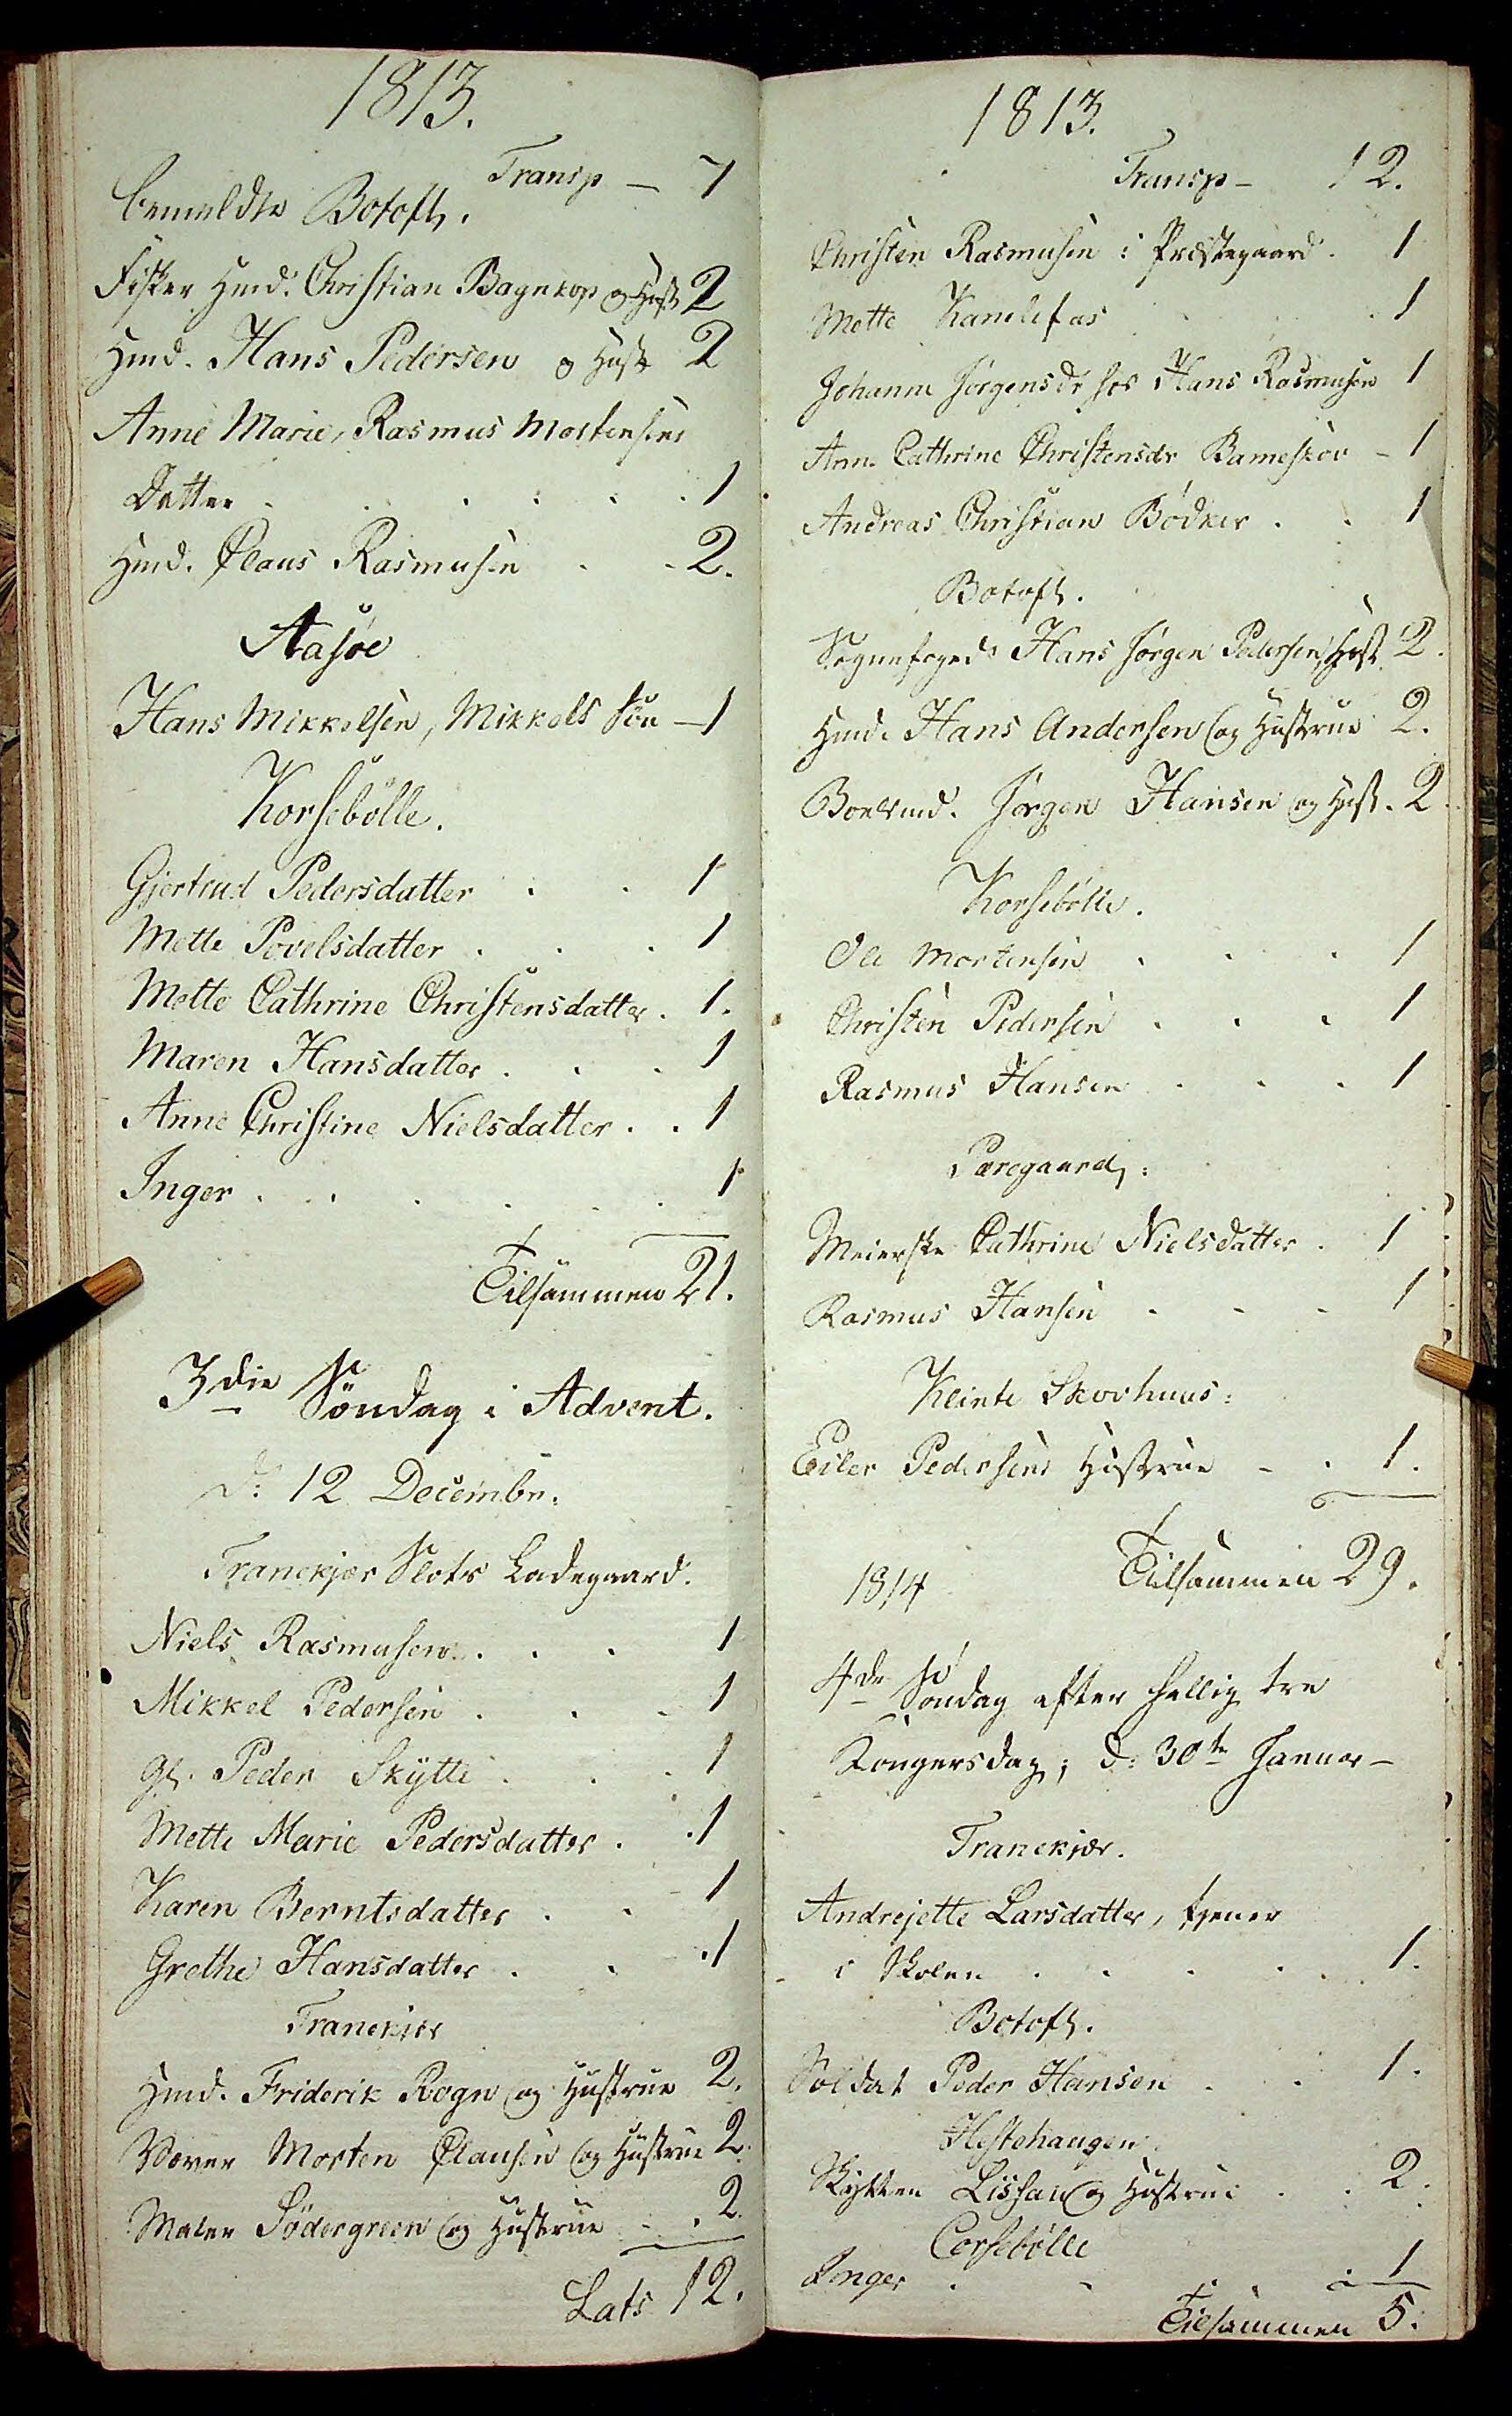1813.
Transp - 7
bemeldte Botoft.
Fisker Hmd. Christian Bagnkop og Hust 2
Hmd. Hans Pedersen og Hust 2
Anne Marie, Rasmus Mortensens
Datter . . . . . - 1
Hmd. Claus Rasmusen . . 2.
Aasøe
Hans Mikkelsen, Michels Søn - 1
Korsebølle.
Gjerten Pedersdatter . . 1
Mette Povelsdatter . . . 1
Mette Cathrine Christensdatter . 1.
Maren Hansdatter . . . 1
Anne Christine Nielsdatter . . 1
Inger . . . . . . 1
Tilsammen 21.
3die Søndag i Advent:.
d: 12 Decembr.
Tranekjær Slots Ladegaard.
Niels Rasmussen . . . 1
Mikkel Pedersen . . . 1
gl. Peder Skytte . . . 1
Mette Marie Pedersdatter . . 1
Karen Berntsdatter . . 1
Grethe Hansdatter . . . 1
Tranekiær
Hmd Friderik Rogn og Hustrue 2.
Væver Morten Clausen og Hustrue 2
Maler Sødergreen og Hustrue . . 2
Lats 12.
1813.
Transp - 12.
Christen Rasmusen i Præstegaard . 1
Mette Kanelefas . . . 1
Johanne Jørgensdr hos Hans Rasmusen 1
Anne Cathrine Christensdr Bameskov - 1
Andreas Christian Bødker . . 1
Botoft.
Sognefoged Hans Jørgen Pedersen Hust 2
Hmd. Hans Andersen og Hustrue 2.
Boelsmd. Jørgen Hansen og Hust . 2.
Korsebølle.
Ole Mortensen . . . 1
Christen Pedersen . . . 1
Rasmus Hansen . . . 1
Pæregaard:
Meierske Cathrine Nielsdatter . 1.
Rasmus Hansen . . . 1
Klinte Skovhuus:
Eiler Pedersens Hustrue . . 1.
Tilsammen 29.
1814
4de Søndag efter hellig tre
Kongersdag; d: 30te Januar.
Tranekjær.
Andrejette Larsdatter, tjener
i Skolen . . . . . 1.
Botoft.
Soldat Peder Hansen . . 1.
Hestehaugen
Skytten Lissau og Hustru 2.
Corsebølle
Inger . . . . . 1
Tilsammen 5.
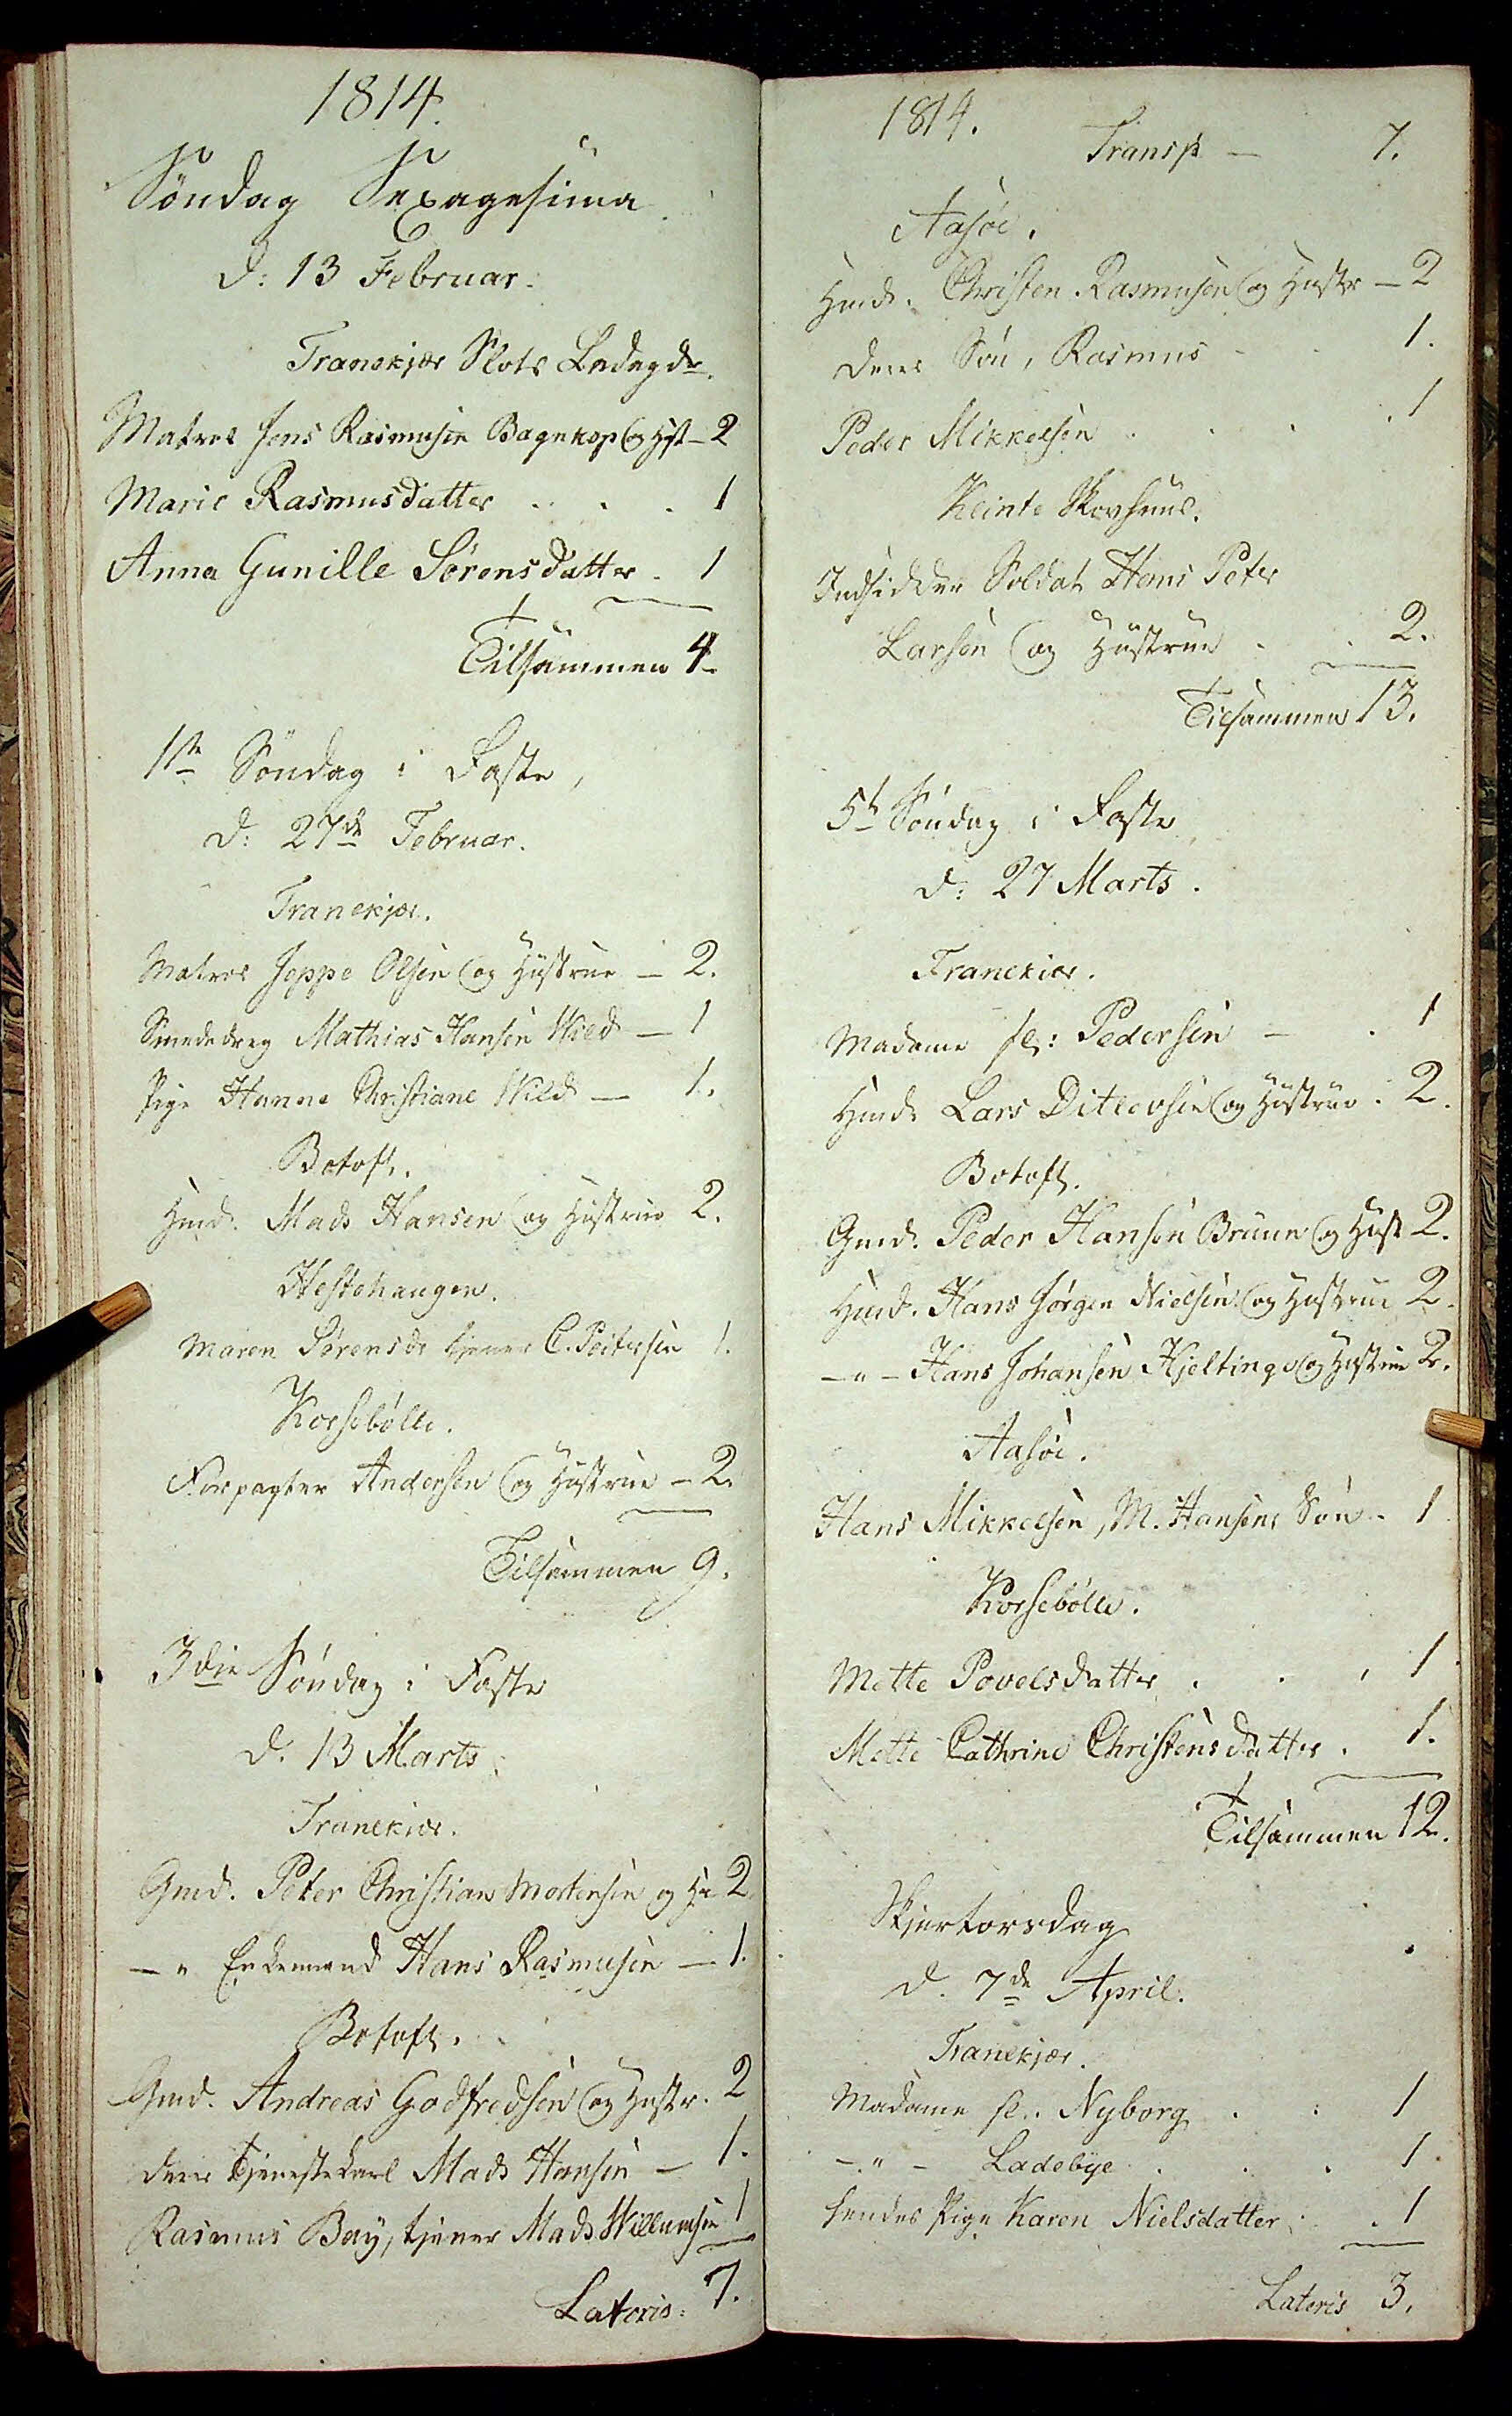1814.
Søndag Sexagesima
d: 13 Februar:
Tranekjær Slots Ladegdr.
Matros Jens Rasmussen Bagnkop og Hst - 2
Marie Rasmusdatter . . . 1
Anna Gunille Sørensdatter . 1
Tilsammen 4.
1ste Søndag i Faste
d: 27de Februar.
Tranekjær.
Matros Jeppe Olsen og Hustrue - 2.
Smededreng Mathias Hansen Wild - 1
Pige Hanne Christiane Wild - 1.
Botoft
Hmd. Mads Hansen og Hustrue 2.
Hestehaugen.
Maren Sørensdr tjener C. Peitersen 1.
Korsebølle.
Forpagter Andersen og Hustrue - 2.
Tilsammen 9.
3die Søndag i Faste
d. 13 Marts
Tranekiær.
Gmd. Peter Christian Mortensen og Hu 2
-"- Enkemand Hans Rasmusen - 1.
Botoft.
Gmd. Andreas Godsfredsen og Hustr . 2
deres Tjenestekarl Mads Hansen - 1.
Rasmus Bay tjener Mads Willumsen 1
Lateris: 7.
1814.
Transp - 7.
Aasøe.
Hmd. Christen Rasmusen og Hustr - 2
deres Søn, Rasmus . . 1.
Peder Mikkelsen . . . . 1
Klinte Skovhuus.
Indsidder Soldat Hans Peter
Larsen og Hustrue . . 2.
Tilsammen 13.
5te Søndag i Faste
d: 27 Marts.
Tranekiær.
Madame sl: Pedersen - . 1
Hmd. Lars Ditlevsen og Hustrue . 2.
Botoft.
Gmd. Peder Hansen Bruun og Hust 2.
Hmd. Hans Jørgen Nielsens og Hustrue 2.
-"- Hans Johansen Hjeltinge og Hustrue 2.
Aasøe.
Hans Mikkelsen, M. Hansens Søn . 1
Korsebølle.
Mette Povelsdatter . . . 1
Mette Cathrine Christensdatter . 1.
Tilsammen 12.
Skjertorsdag
d. 7de April.
Tranekjær
Madame sl. Nyborg . . 1
-"- Ladebye . . . 1.
hendes Pige Karen Nielsdatter . . 1
Lateris 3.